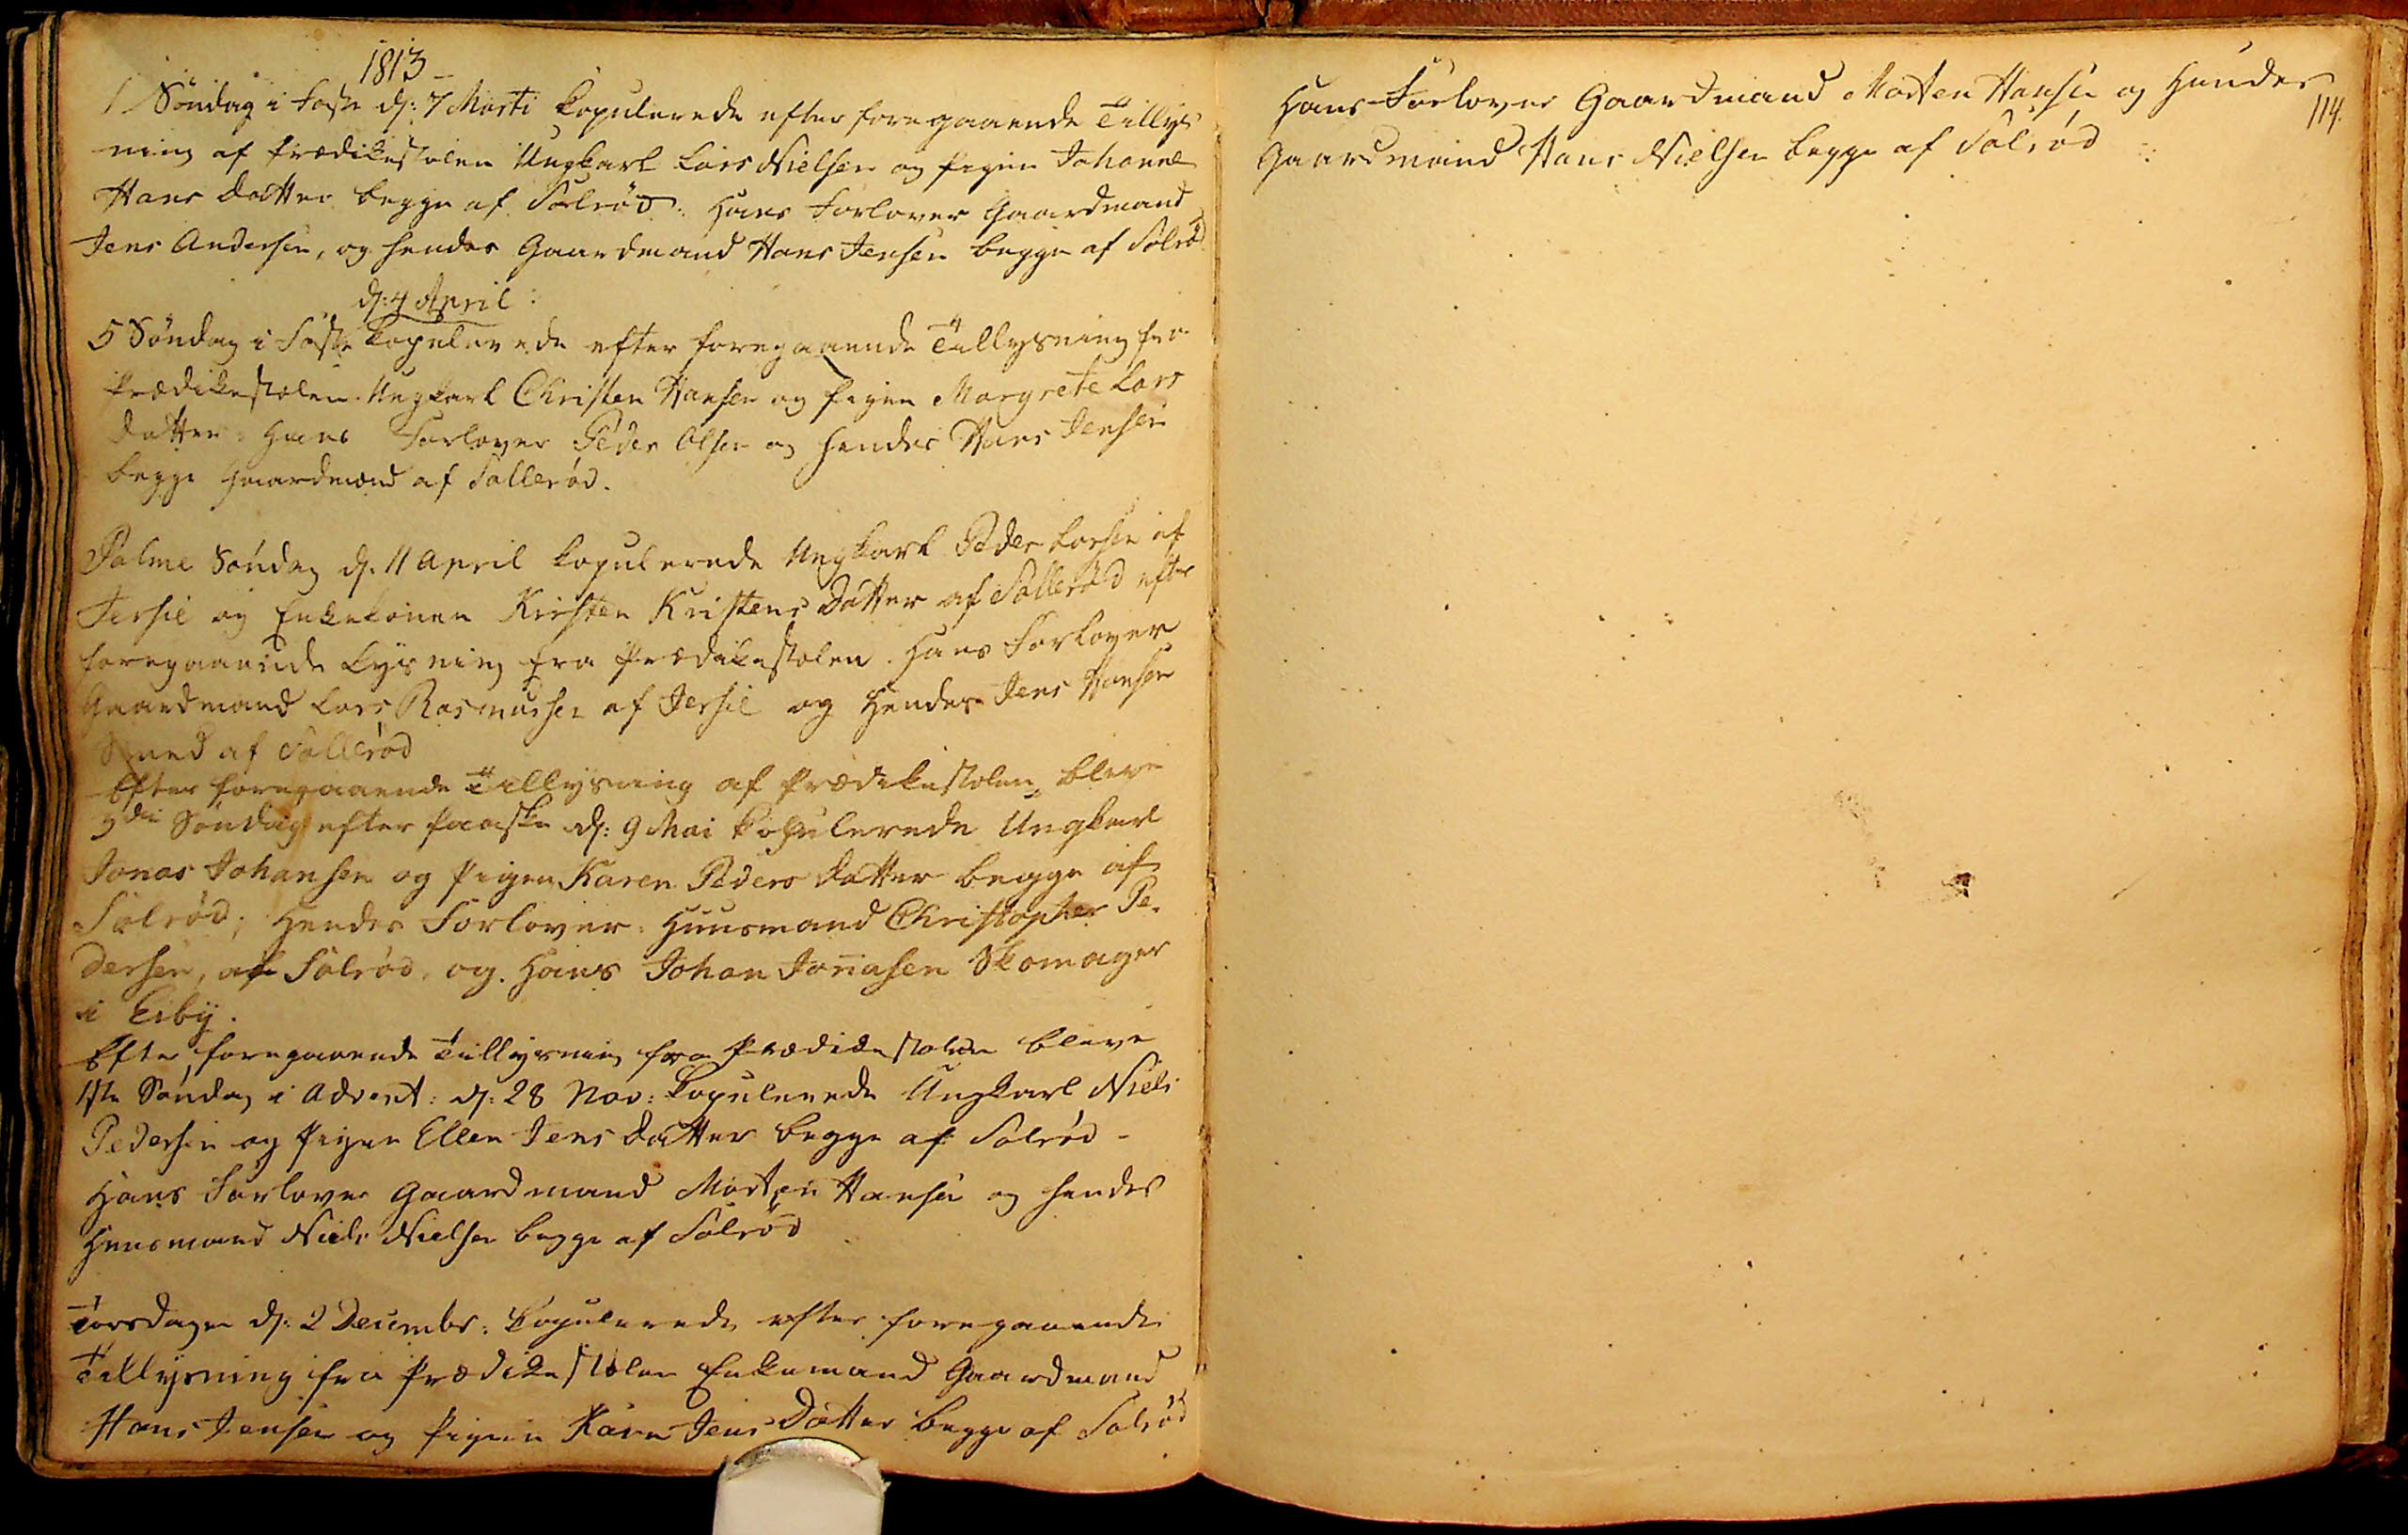1813
1 Søndag i Faste d: 7 Marti Kopulerede efter foregaaende Tillys
ning af Prædikestolen Ungkarl Lars Nielsen og Pigen Johanne
Hans Datter begge af Solrød. Hans Forlover Gaardmand
Jens Andersen, og hendes Gaardmand Hans Jensen begge af Solrød
d: 4 April.
5 Søndag i Faste Kopulerede efter foregaaende Tillysning fra
Prædikestoeln Ungkarl Christen Hansen og Pigen Margrete Lars
Datter: Hans Forlover Peder Olsen og hendes Hans Jensen
begge Gaardmænd af Sollerød.
Palme Søndag d: 11 April Kopulerede Ungkarl Peder Larsen af
Jersie og Enkekonen Kirsten Kristens Datter af Sollerød efter
foregaaende Lysning fra Prædikestolen. Hans Forlover
Gaardmand Lars Rasmussen af Jersie og Hendes Jens Hansen
Smed af Sollerød
Efter foregaaende Tillysning af Prædikestolen blev
3die Søndag efter Paaske d: 9 Mai Kopulerede Ungkarl
Jonas Johansen og Pigen Karen Peders Datter begge af
Solrød; Hendes Forlover Huusmand Christopher Pe¬
dersen, af Solrød, og Hans Johan Jonasen Skomager
i Eiby.
Efter foregaaende Tillysning fra Prædikestolen blev
1ste Søndag i Advent: d: 28 Nov: Kopulerede Ungkarl Niels
Pedersen og Pigen Ellen Jens Datter begge af Solrød.
Hans Forlover Gaardmand Morten Hansen og hendes
Huusmand Niels Nielsen begge af Solrød.
Torsdagen d: 2 Decembr: Kopulerede efter foregaaende
Tillysning fra Prædikestolen Enkemand Gaardmand
Hans Jensen og Pigen Karen Jens Datter begge af Solrød
114
Hans Forlover Gaardmand Morten Hansen og Hendes
Gaardmand Hans Nielsen begge af Solrød 
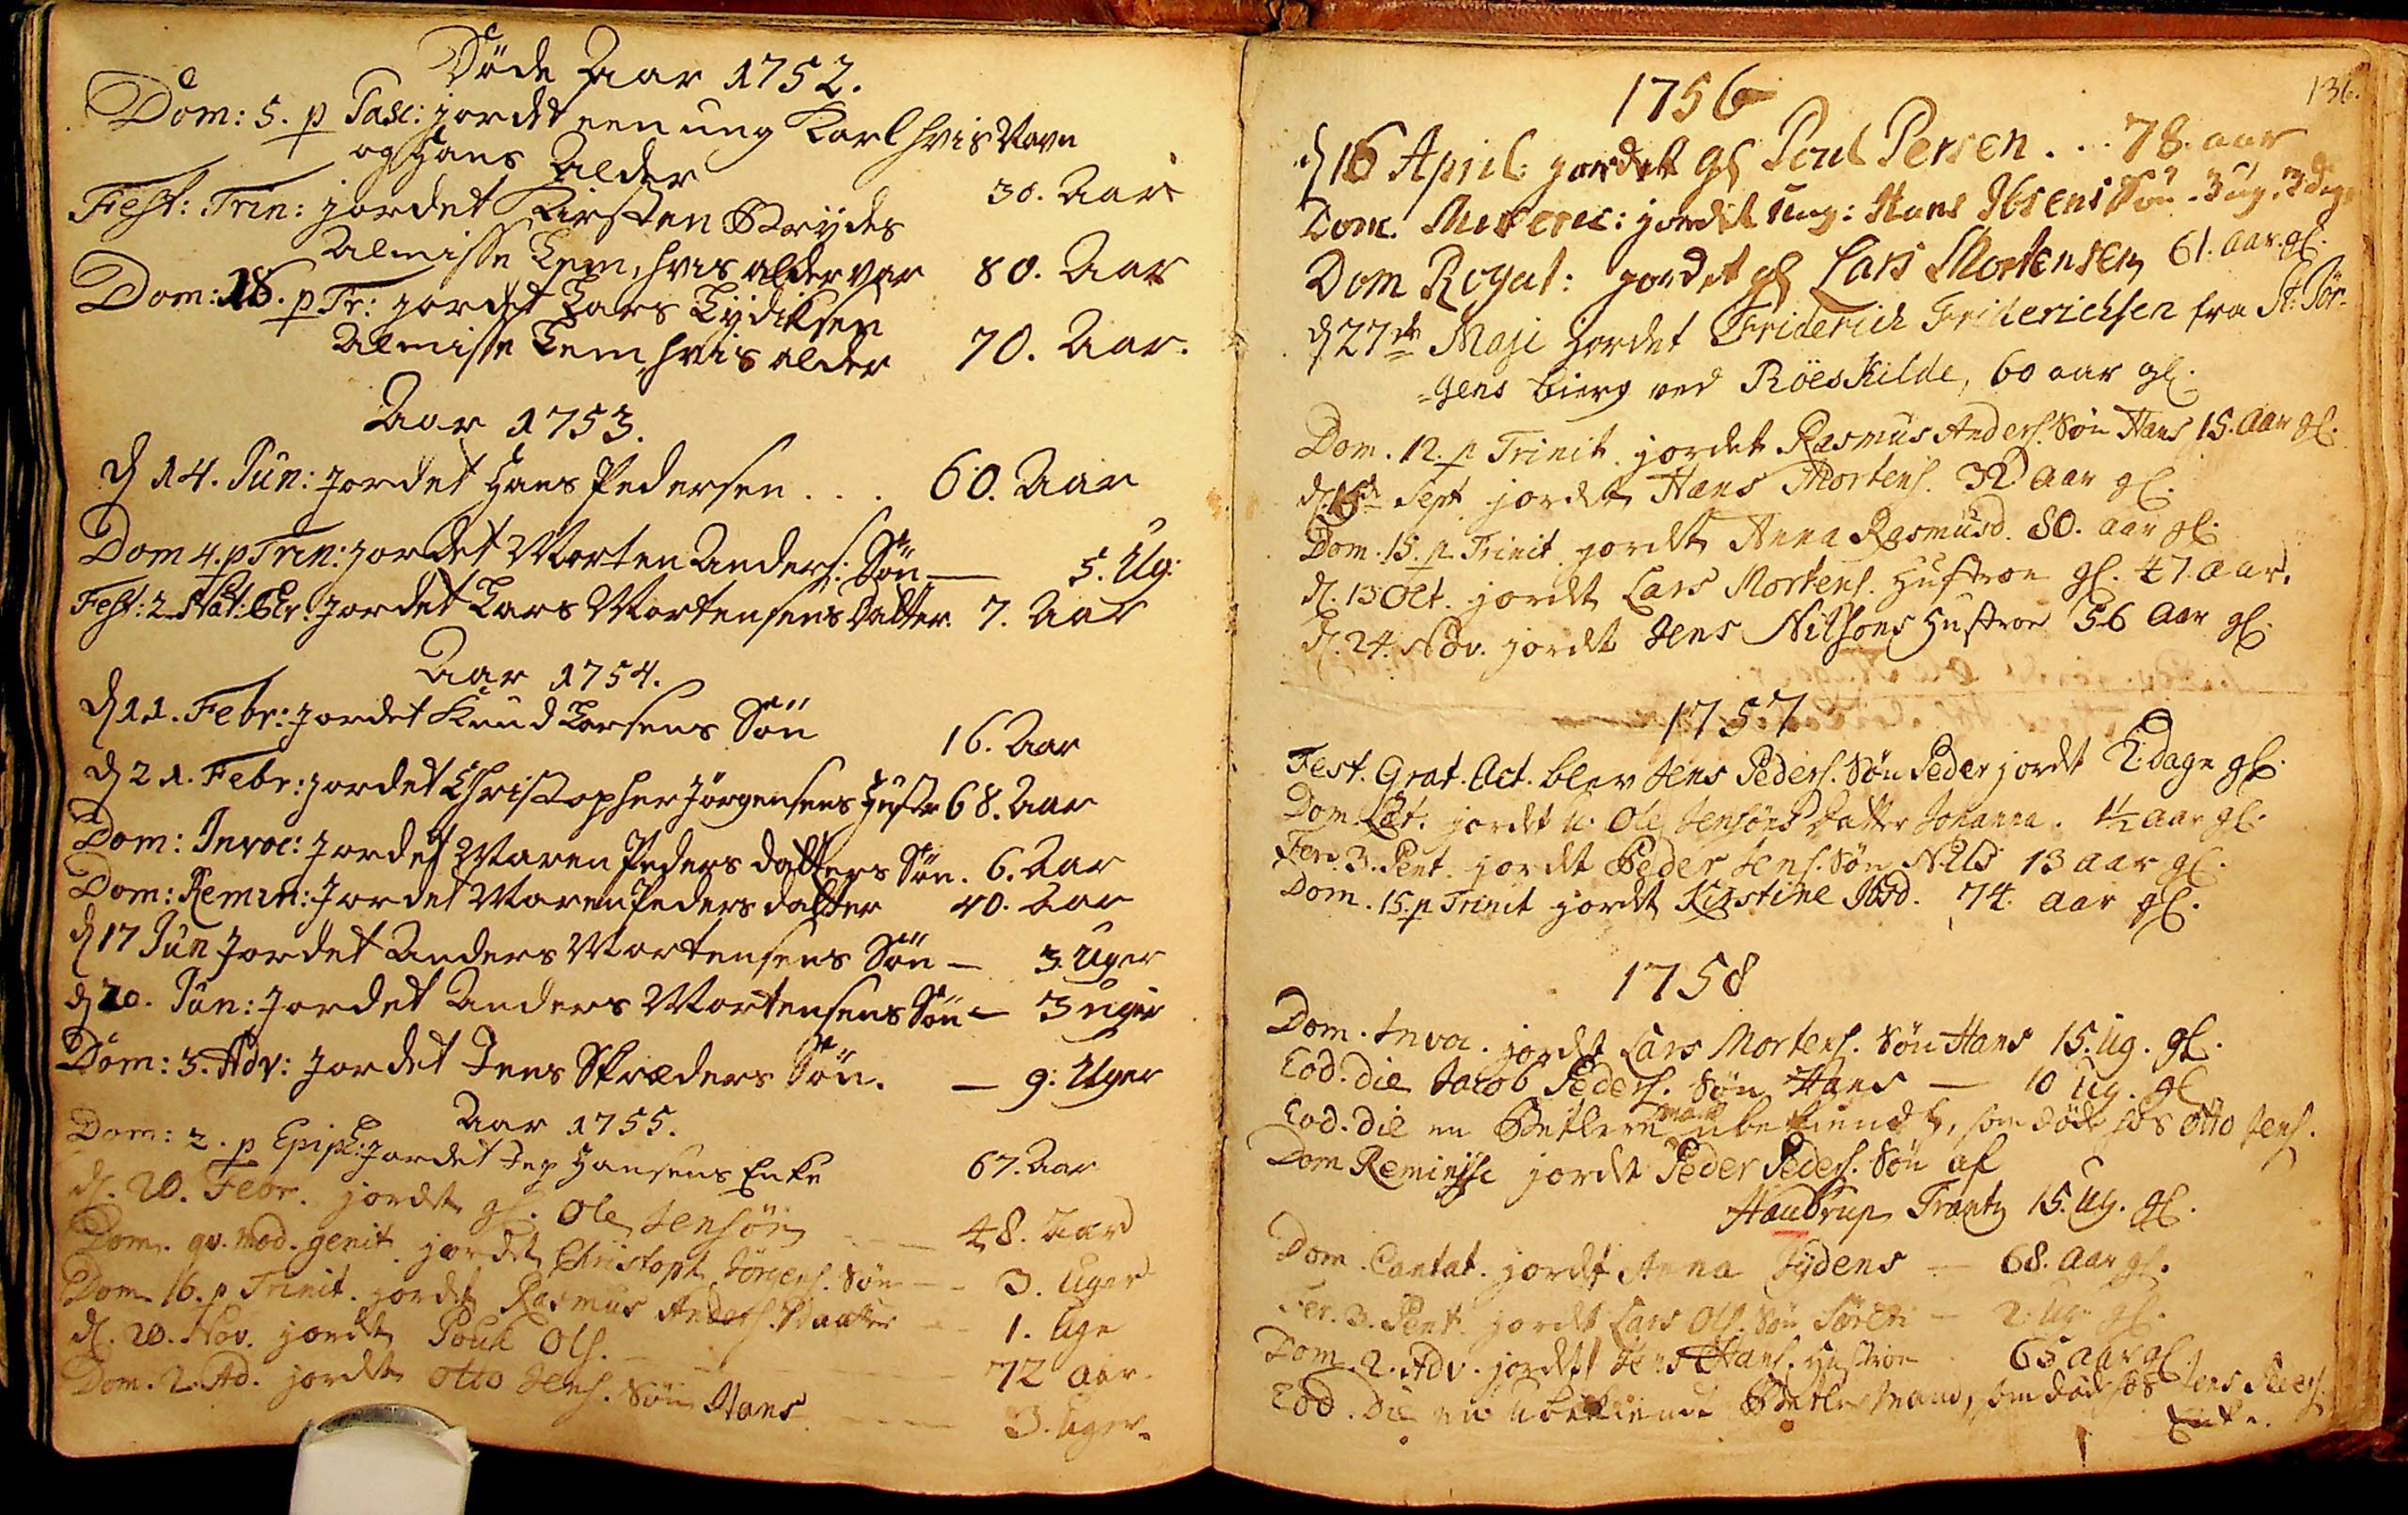Døde Aar 1752.
Dom: 5. p Pasc: jordet een ung Karl hvis Navn
og Hans Alder
30. Aar
Fest: Trin: jordet Kirsten Brydes
Almisse Lem, hvis Alder var 80. Aar
Dom: 18. p Tr: jordet Lars Lydiksen
Almisse Lem, hvis Alder 70. Aar.
Aar 1753.
d 14. Jun: jordet Hans Pedersen . . . 60. Aar
Dom 4. p Trin: jordet Morten Anders: Søn
5.Ug:
Fest: 2. Nat: Chr: jordet Lars Mortensens Datter 7. Aar
Aar 1754.
d 11. Febr: jordet Knud Larsens Søn
16. Aar
d 21. Febr: jordet Christopher Jørgensens Hustr 68. Aar
Dom: Invoc: jordet Maren Pedersdatters Søn. 6. Aar
Dom: Remin: jordet Maren Pedersdatter 40. Aar
d 17 Jun jordet Anders Mortensens Søn - 3. Uger
d 20. Jun: jordet Anders Mortensens Søn - 3 Uger
Dom: 3. Adv: jordet Jens Skræders Søn.
9. Uger
Aar 1755.
Dom: 2. p Epiph: jordet Jep Hansens Enke
67. Aar
d. 20 Febr. jordet gl. Ole Jensøn
48. Aar
Dom. qv.mod.genit. jordet Christoph. Jørgens. Søn
3. Uger
Dom 16. p Trinit. jordet Rasmus Anders. Daatter
1. Uge
d. 20. Nov. jordet Poul Ols.
72 Aar.
Dom. 2. Ad. jordet Otto Jens. Søn Hans
3. Uger
136.
1756
d 16 April: jordet gl Poul Persen . . . 78. Aar
Dom. Miserec: jordet Ung: Hans Ibsens Søn 3 ug. 3 dage
Dom Rogat: jordet gl Lars Mortensen 61. Aar. gl.
d 27de Maji jordet Friderich Friderichsen fra St: Jör¬
gens Bierg ved RöesKilde, 60 aar gl.
Dom. 12. p Trinit. jordet Rasmus Anders. Søn Hans 15. Aar gl.
d. 19d Sept jordet Hans Mortens. 32 Aar gl.
Dom. 15. p. Trinit. jordet Anna Rasmusd. 80. Aar gl.
d. 13 Oct. jordet Lars Mortens. Hustroe gl. 47 Aar.
d. 24. Nov. jordet Jens Nilsøns Hustroe 56 Aar gl.
1757
Fest. Grat. Act. blev Jens Peders. Søn Peder jordet 2 Dage gl.
Dom. Læt. jordet U. Ole Jensøns Datter Johanna. 1½ Aar gl.
Fer. 3. Pent. jordet Peder Jens. Søn Nils 13 Aar gl.
Dom. 15. p Trinit jordet Kirstine Ibsd. 74. Aar gl.
1758
Dom. Invoc. jordet Lars Mortens. Søn Hans 15. Ug. gl.
Eod. die Jacob Peders. Søn Hans
10 Ug. gl
Mand
Eod. die en Betlere ubekiendt, som døde hos Otto Jens.
Dom Reminisc jordet Peder Peders. Søn af
Haudrup Frantz 15. Ug. gl.
Dom. Cantat. jordet Anna Jydens - 68. Aar gl.
Fer. 3. Pent. jordet Lars Ols. Søn Søren - 2. Ug. gl.
Dom. 2. Adv. jordet Jens Hans. Hustroe 65 Aar gl.
Eod. Die en Ubekiendt Betler Mand, som døde hos Jens Peders.
Enke.